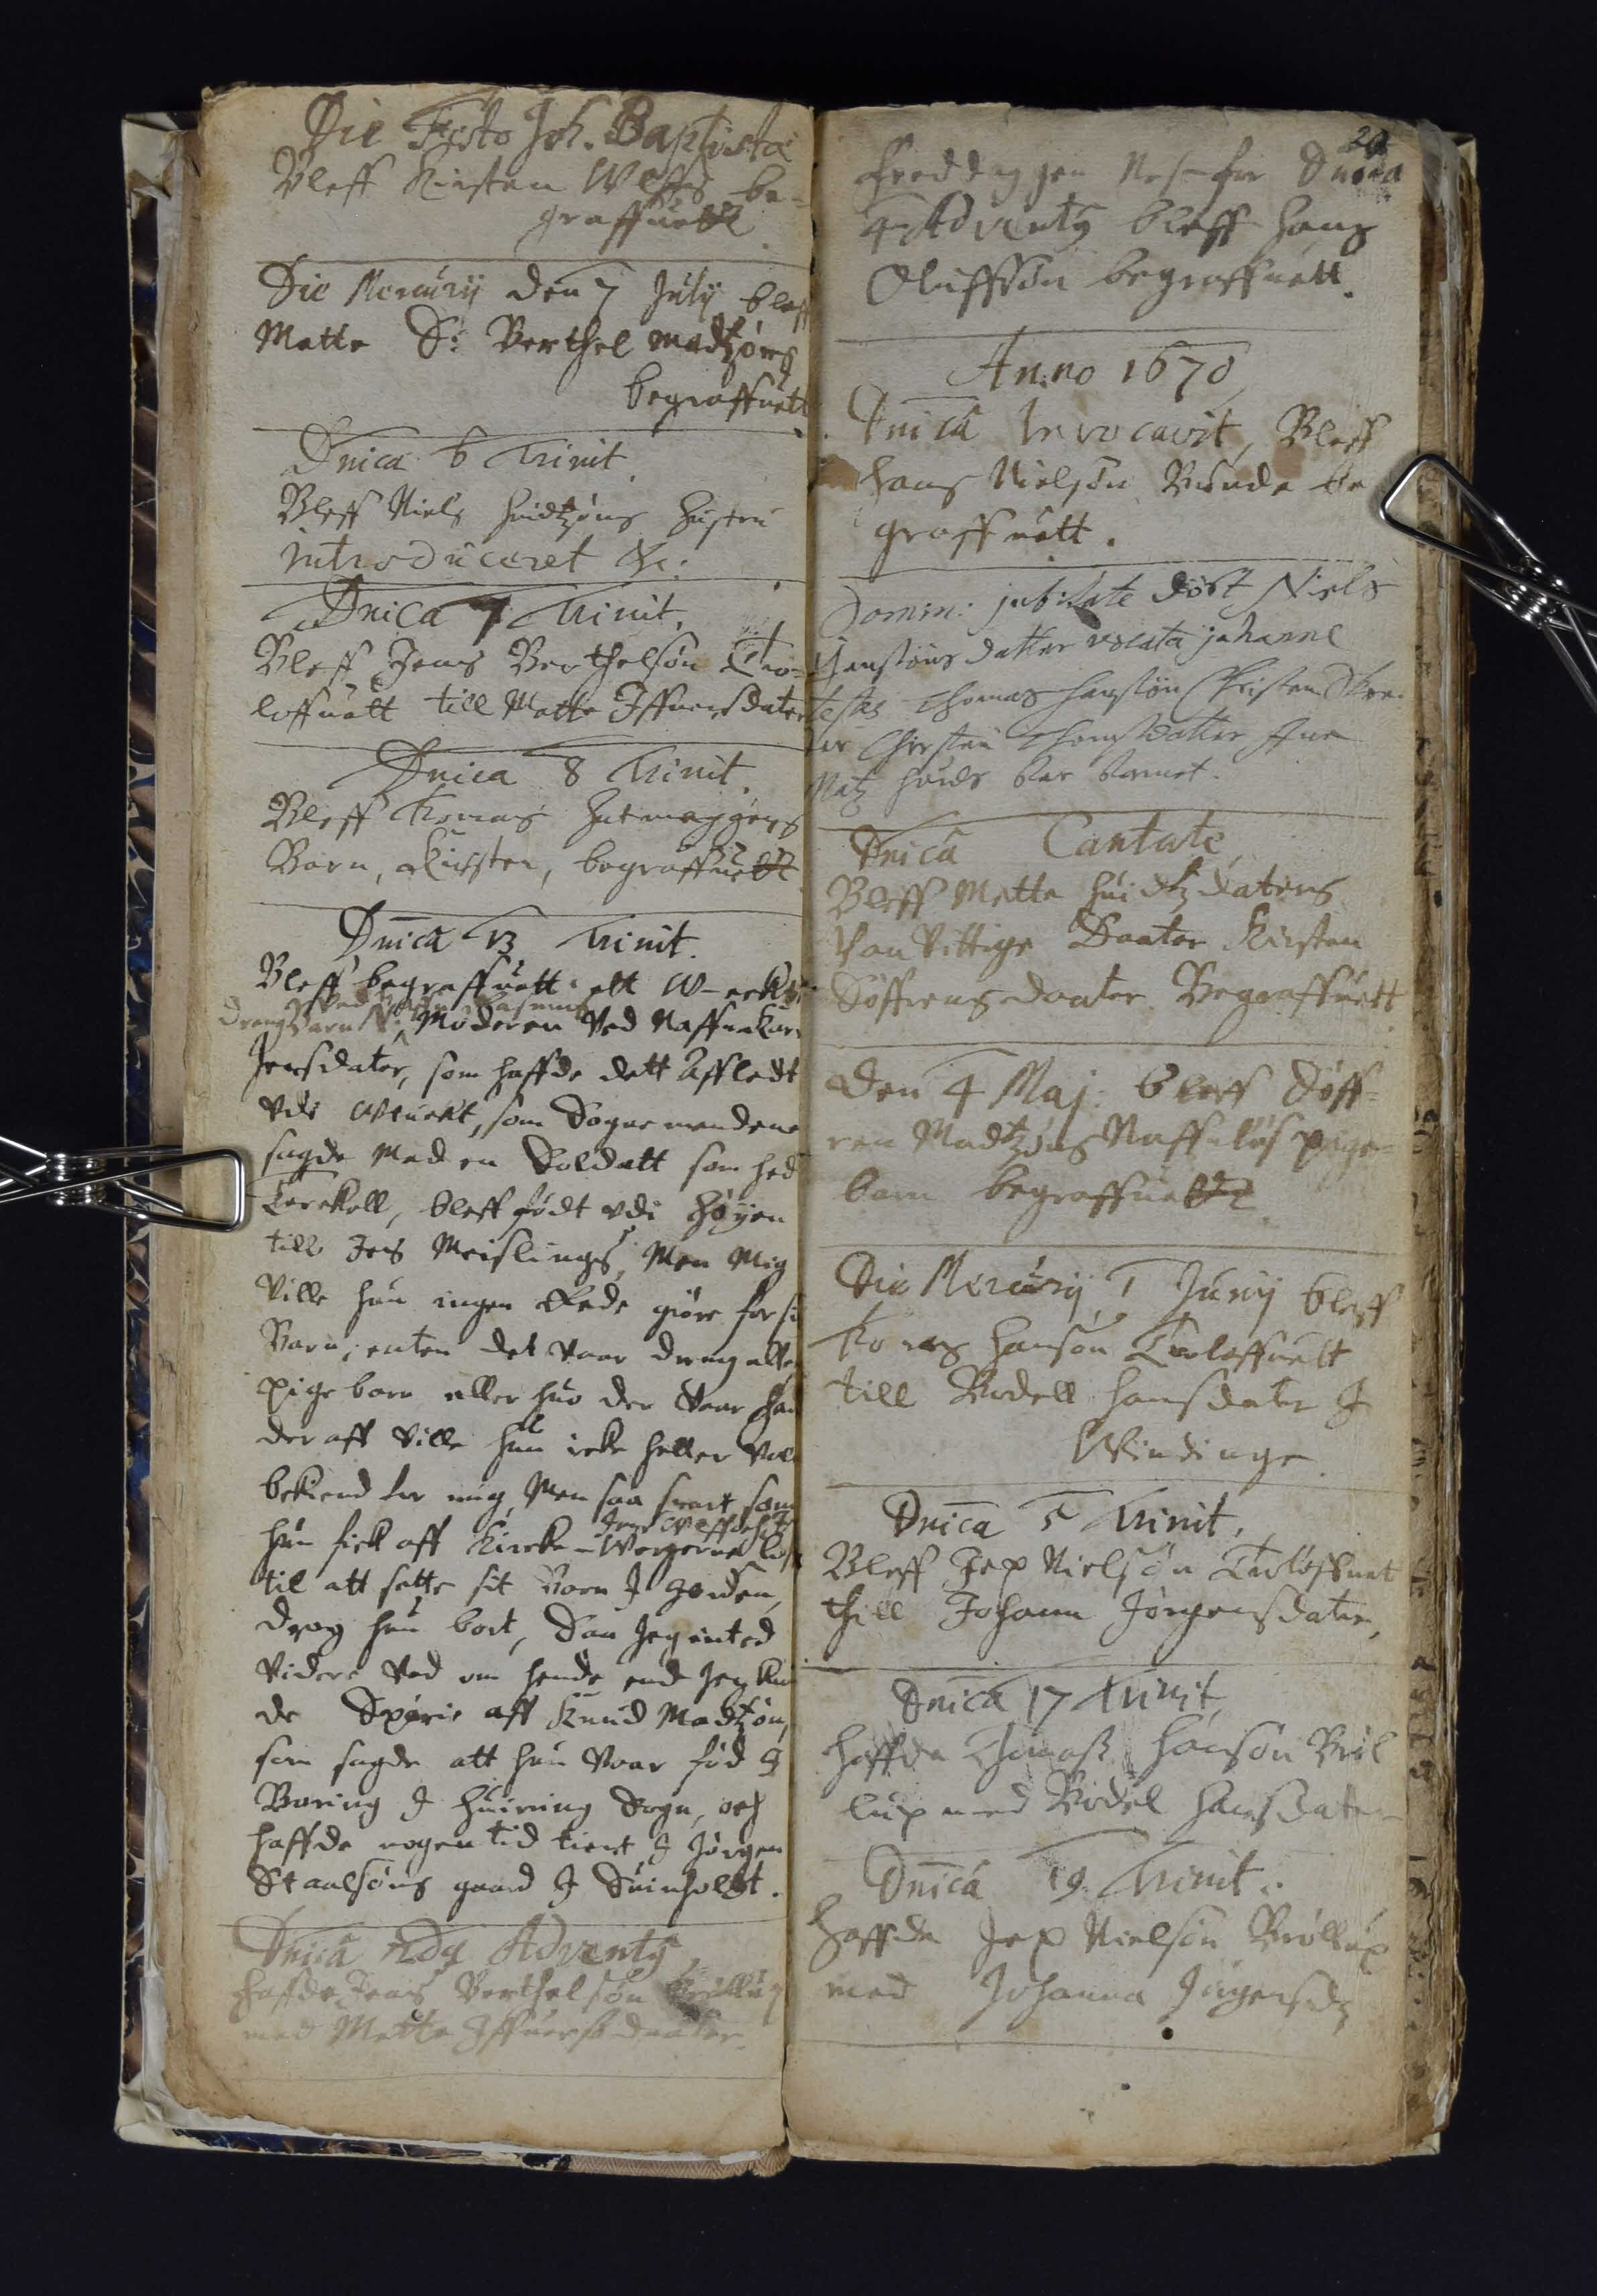Die Festo Joh. Baptista
Bleff Kirsten Vlffes be¬
graffuett
Die Mercurij den 7 Julij bleff
Mette S: Berthel Madtzøns
begraffuett
Dnica 6 Trinit
Bleff Niels Hvidtzøns Hustru
introduceret
Dnica 7 Trinit
Bleff Jens Berthelsøn Tro¬
loffuett till Mette Iffuersdater
Dnica 8 Trinit
Bleff Thomas Hatmaggers
Barn Kiersten begraffuett
Dnica 13 Trinit
Bleff begraffuett ett V-eckte
ved naffn Rasmus
drenge Barn N. Moderen ved naffn
Jensdater, som haffde dett Affledt
vdi Vtuckt, som Sogne mændene
sagde Med en Soldatt som hed
Terckell, bleff født vdi Høyen
til Jes Meislings, Men Mig
ville hun ingen Rede giøre for si 
Barn, enten det Vaar dreng eller
pige barn eller huo der vaar Fa 
der aff ville hun icke heller v##
bekiend for mig, Men saa snart som
Jens Vlff## och Je##
hun fick aff Kircke-Wergerne lof 
til att sette sit Barn I jorden,
drog hun bort, Saa jeg inted
videre ved om hende end Jeg k 
de Spørge aff Knud Madtzøn,
som sagde att hun vaar fød I
Boring I Huiring Sogn, och
haffde nogen tid tient I Jørgen
Staalsøns gaard I Suinholdt.
Dnica 2da Adventij
haffde Jens Berthelsøn Brøllup
med Mette Iffuersdaater.
20
Freddaggen nest for Dnica
4 Adventq Bleff Hans
Oluffsøn begraffuett
Anno 1670
Dnica Invocavit, Bleff
Hans Nielsøn Bunde be
graffuett.
Domin. Jubilate. døbt Niels
Jensøns datter ##ta Johanne
Testes. Thomas Hansøn. Christen Skre¬
der. Chirsten Thomasdatter Ane
Matz Hvids bar barnet
Dnica Cantate
Bleff Mette Hvidtzdaters
Van Vittige Daater Kirsten
Søffrensdaater Begraffuett
den 4 Maj. bleff Søff¬
ren Madtzøns Naffnløs pige¬
barn begraffuett
Die Mercurij. 1 Junij bleff
Thomas Hansøn Troloffuett
till Bodell Hansdater I
Windinge.
Dnica 5 Trinit.
Bleff Jep Nielsøn Troloffuet
thill Johanne Jørgensdater
Dnica 17 Trinit
Haffde Thomass Hansøn Brøl
lup med Bodel Hansdater
Dnica 19 Trinit.
Haffde Jep Nielsøn Brøllup
med Johanna Jørgensd
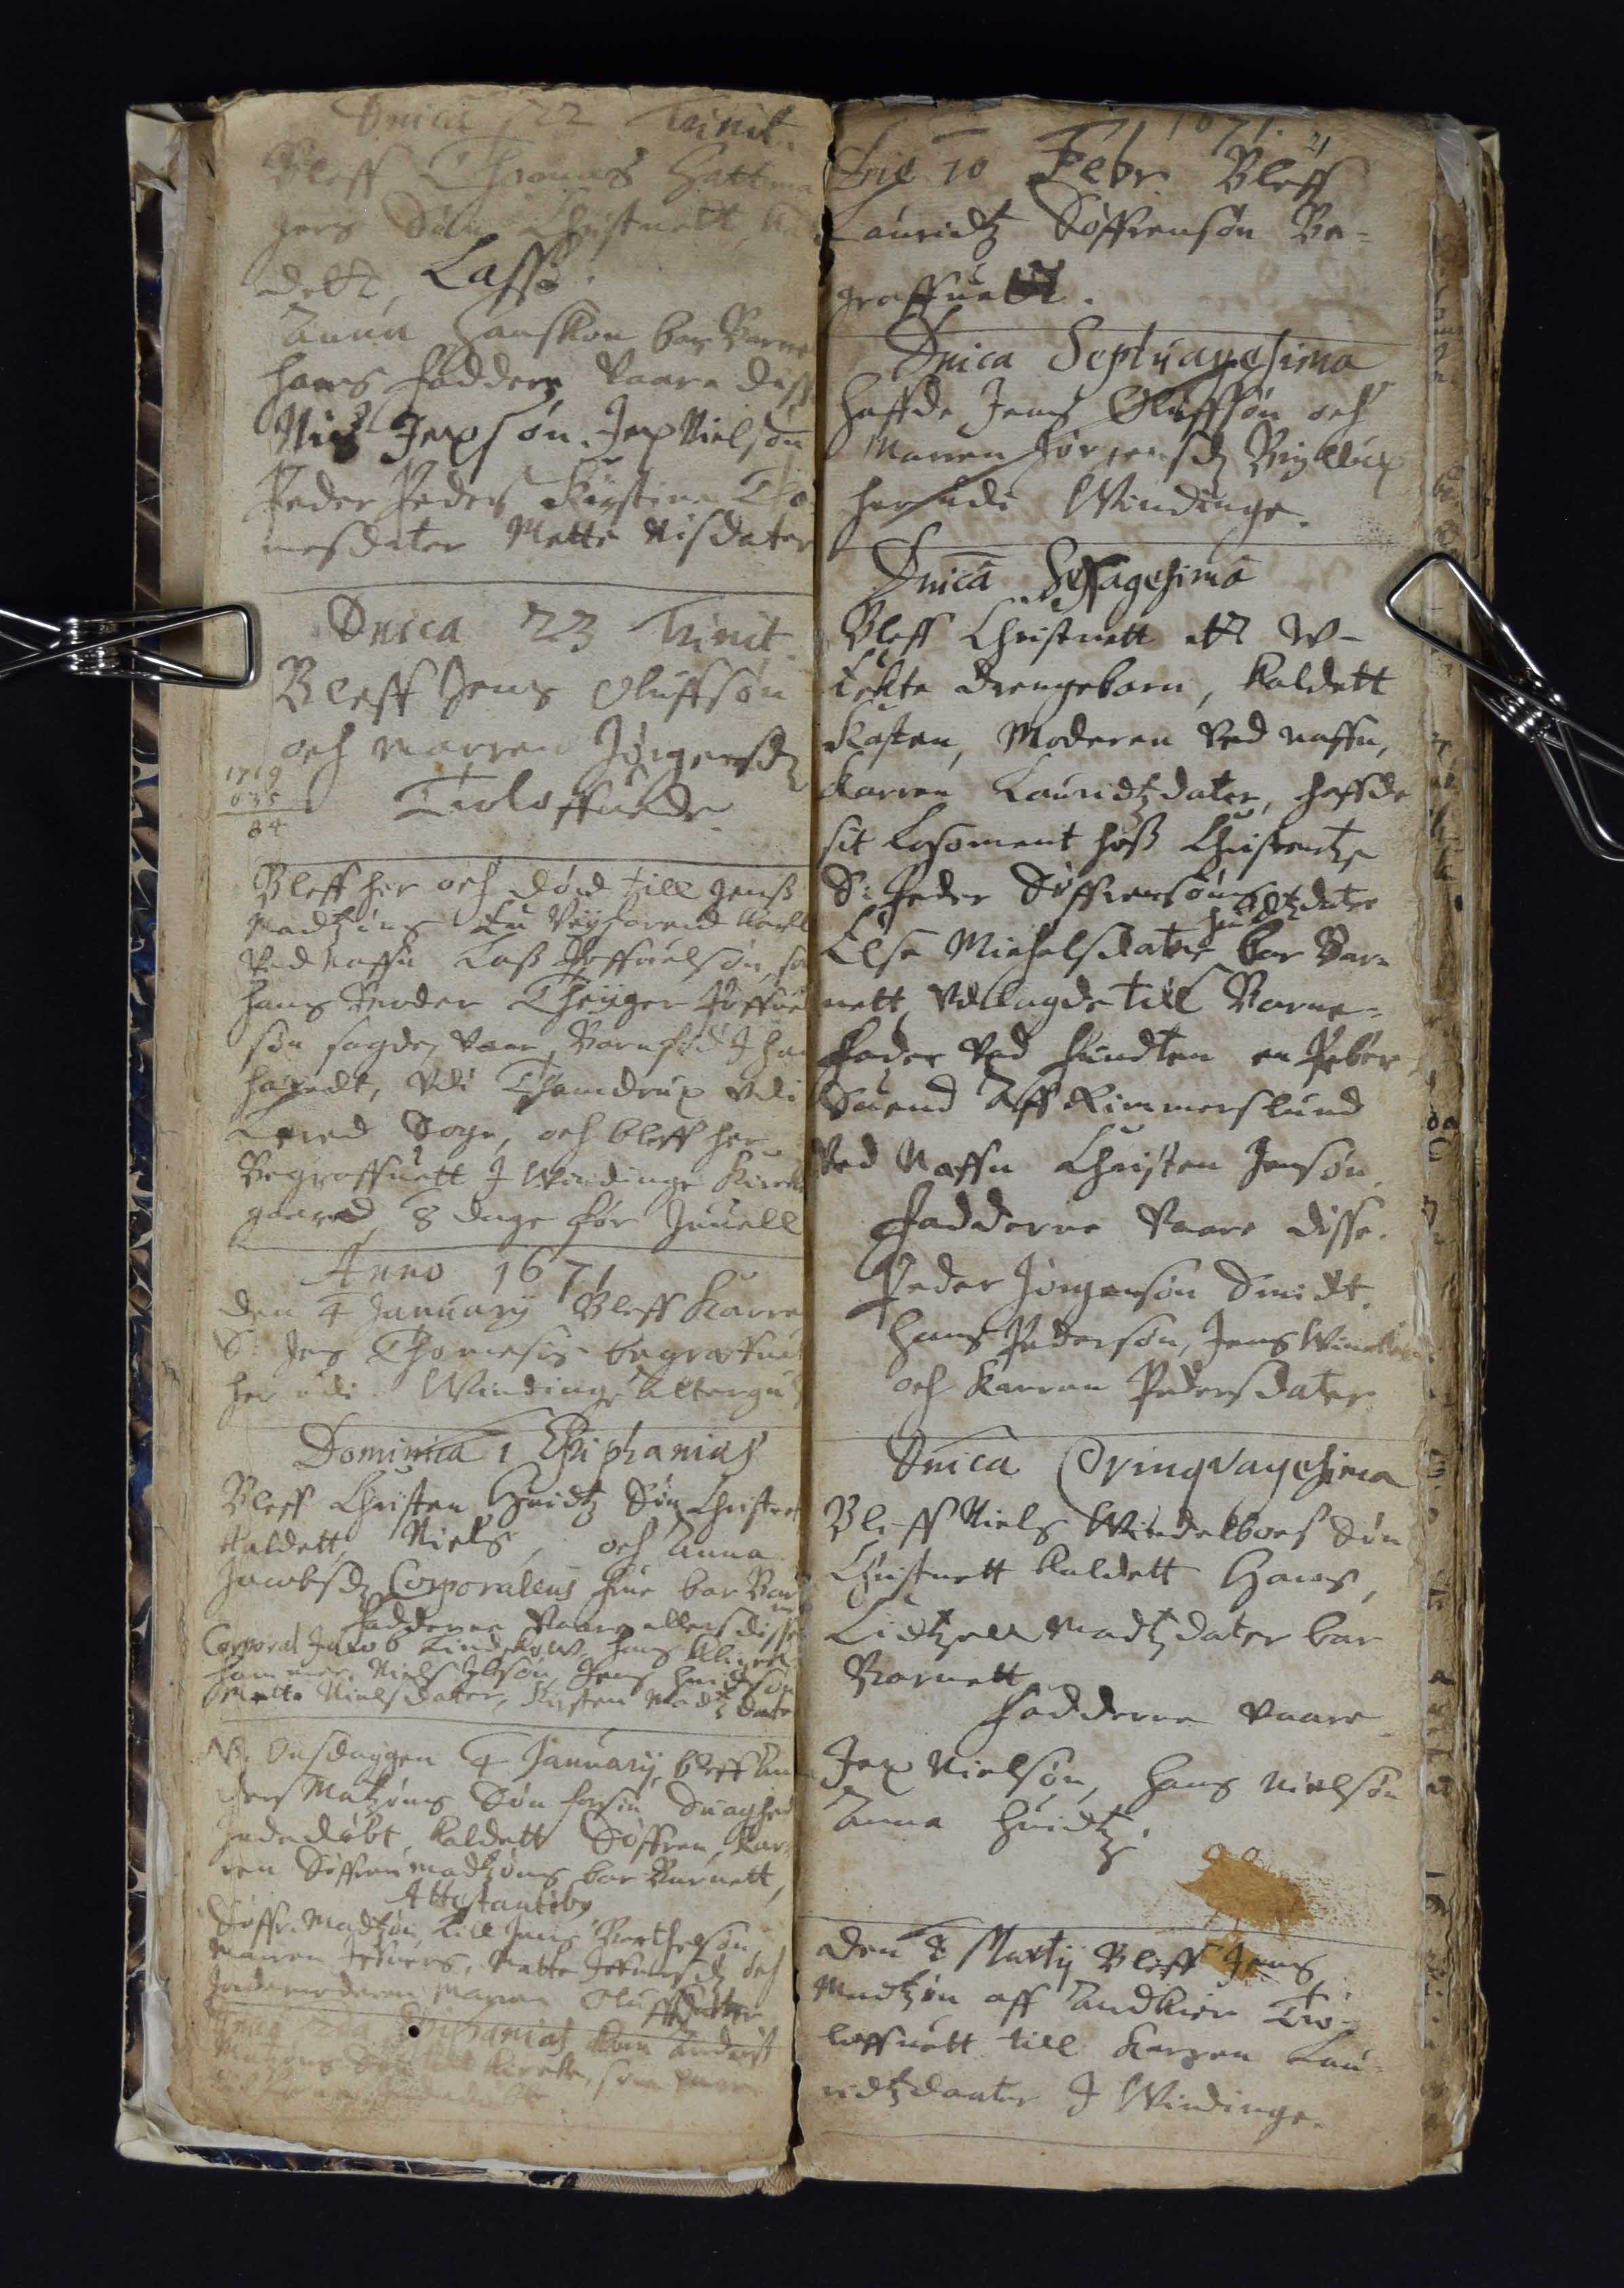Dnica 22 Trinit.
Bleff Thomas Hattma
gers Søn Christnet ka 
dett Lasse.
Anna Hans kon bar Barnet
Hans faddere vaare diss 
Nis Jepsøn. Jep Nielsøn
Peder Peder Kirstine Tho¬
masdater Mette Nisdater
Dnica 23 Trinit.
Bleff Jens Oluffsøn
och Marren Jørgensd
Troloffuede.
Bleff her och død till Jenss
Madtzøns En Veijfarend karll
ved naffn Lass Poffuelsøn, som
hans Broder Theijger Poffue 
søn sagde vaar Barnfød I ha 
vdi Thamdrup vdi
Begraffuett I Windinge Kircke
Sogn, och bleff her
gaard 8 dage før Juuell
Anno 1671
den 4 Januarij. Bleff Karren
S: Jes Thomesis begraffuet 
her udi Windinge Altergul 
Dominica 1 Epiphanias
Bleff Christen Hvidtz Søn Christnet
kaldett NIELS, och Anna
Jacobs Corporalens Frue bar Bar
net
Faddere vaare ellers disse
Corporal Jacob Lindskow## Hans Klieck
hammer. Niels ##søn, Jens Hvidsøn
Mette Nielsdater. Kirsten Madtzdater
NB. Onsdagen 4 Januarij. bleff An
ders Madtzøns Søn for sin Suaghed
Indedøbt, kaldett Søffren, Kar¬
ren Søffren Madtzøns bar Barnett
Attestantiby
Søffren Madtzøn. Lill Jens Berthelsøn
Marren Iffuers, Mette Iffuersd. och
Jordemoderen Marren Oluffsdatter
Dnica 2da Epiphanias kom Anderss
Madtzøns Søn till Kierke, som
## ## ## Indedøbt
21
Die 10 Febr. Bleff
Lauridtz Søffrensøn Be¬
graffuett.
Dnica Septuagesima
Haffde Jens Oluffsøn och
Marren Jørgensd Bryllup
her udi Windinge.
Dnica Sexagesima
Bleff Christnett ett W¬
Eckte drengebarn, kaldett
Kasten, Moderen ved naffn
Karren Lauridtzdater, haffde
sit Losoment hoss Christentze
S: Peder Søffrensøns
hindtz dater
Else Michelsdater bar Bar¬
nett Vdlagde till Barne¬
Fader ved Fundten en Peber¬
Svend Aff Rimmerslund
Ved Naffn Christen Jensøn
Faddere Vaare disse
Peder Jørgensøn Smidt.
Hans Pedersøn, Jens
och Karren Pedersdater
Dnica Qvuinqvagesima
Bleff Niels Windelboes Søn 
Christnett kaldett Hans
Cidtzell Madtzdater bar
Barnett
Fadderne vaare
Jep Nielsøn, Hans Nielsøn
Anna Huidtz
den 8 Martij. Bleff Jens
Madtzøn aff Andkier Tro¬
loffuett till Karren Lau¬
ridtzdaater I Windinge